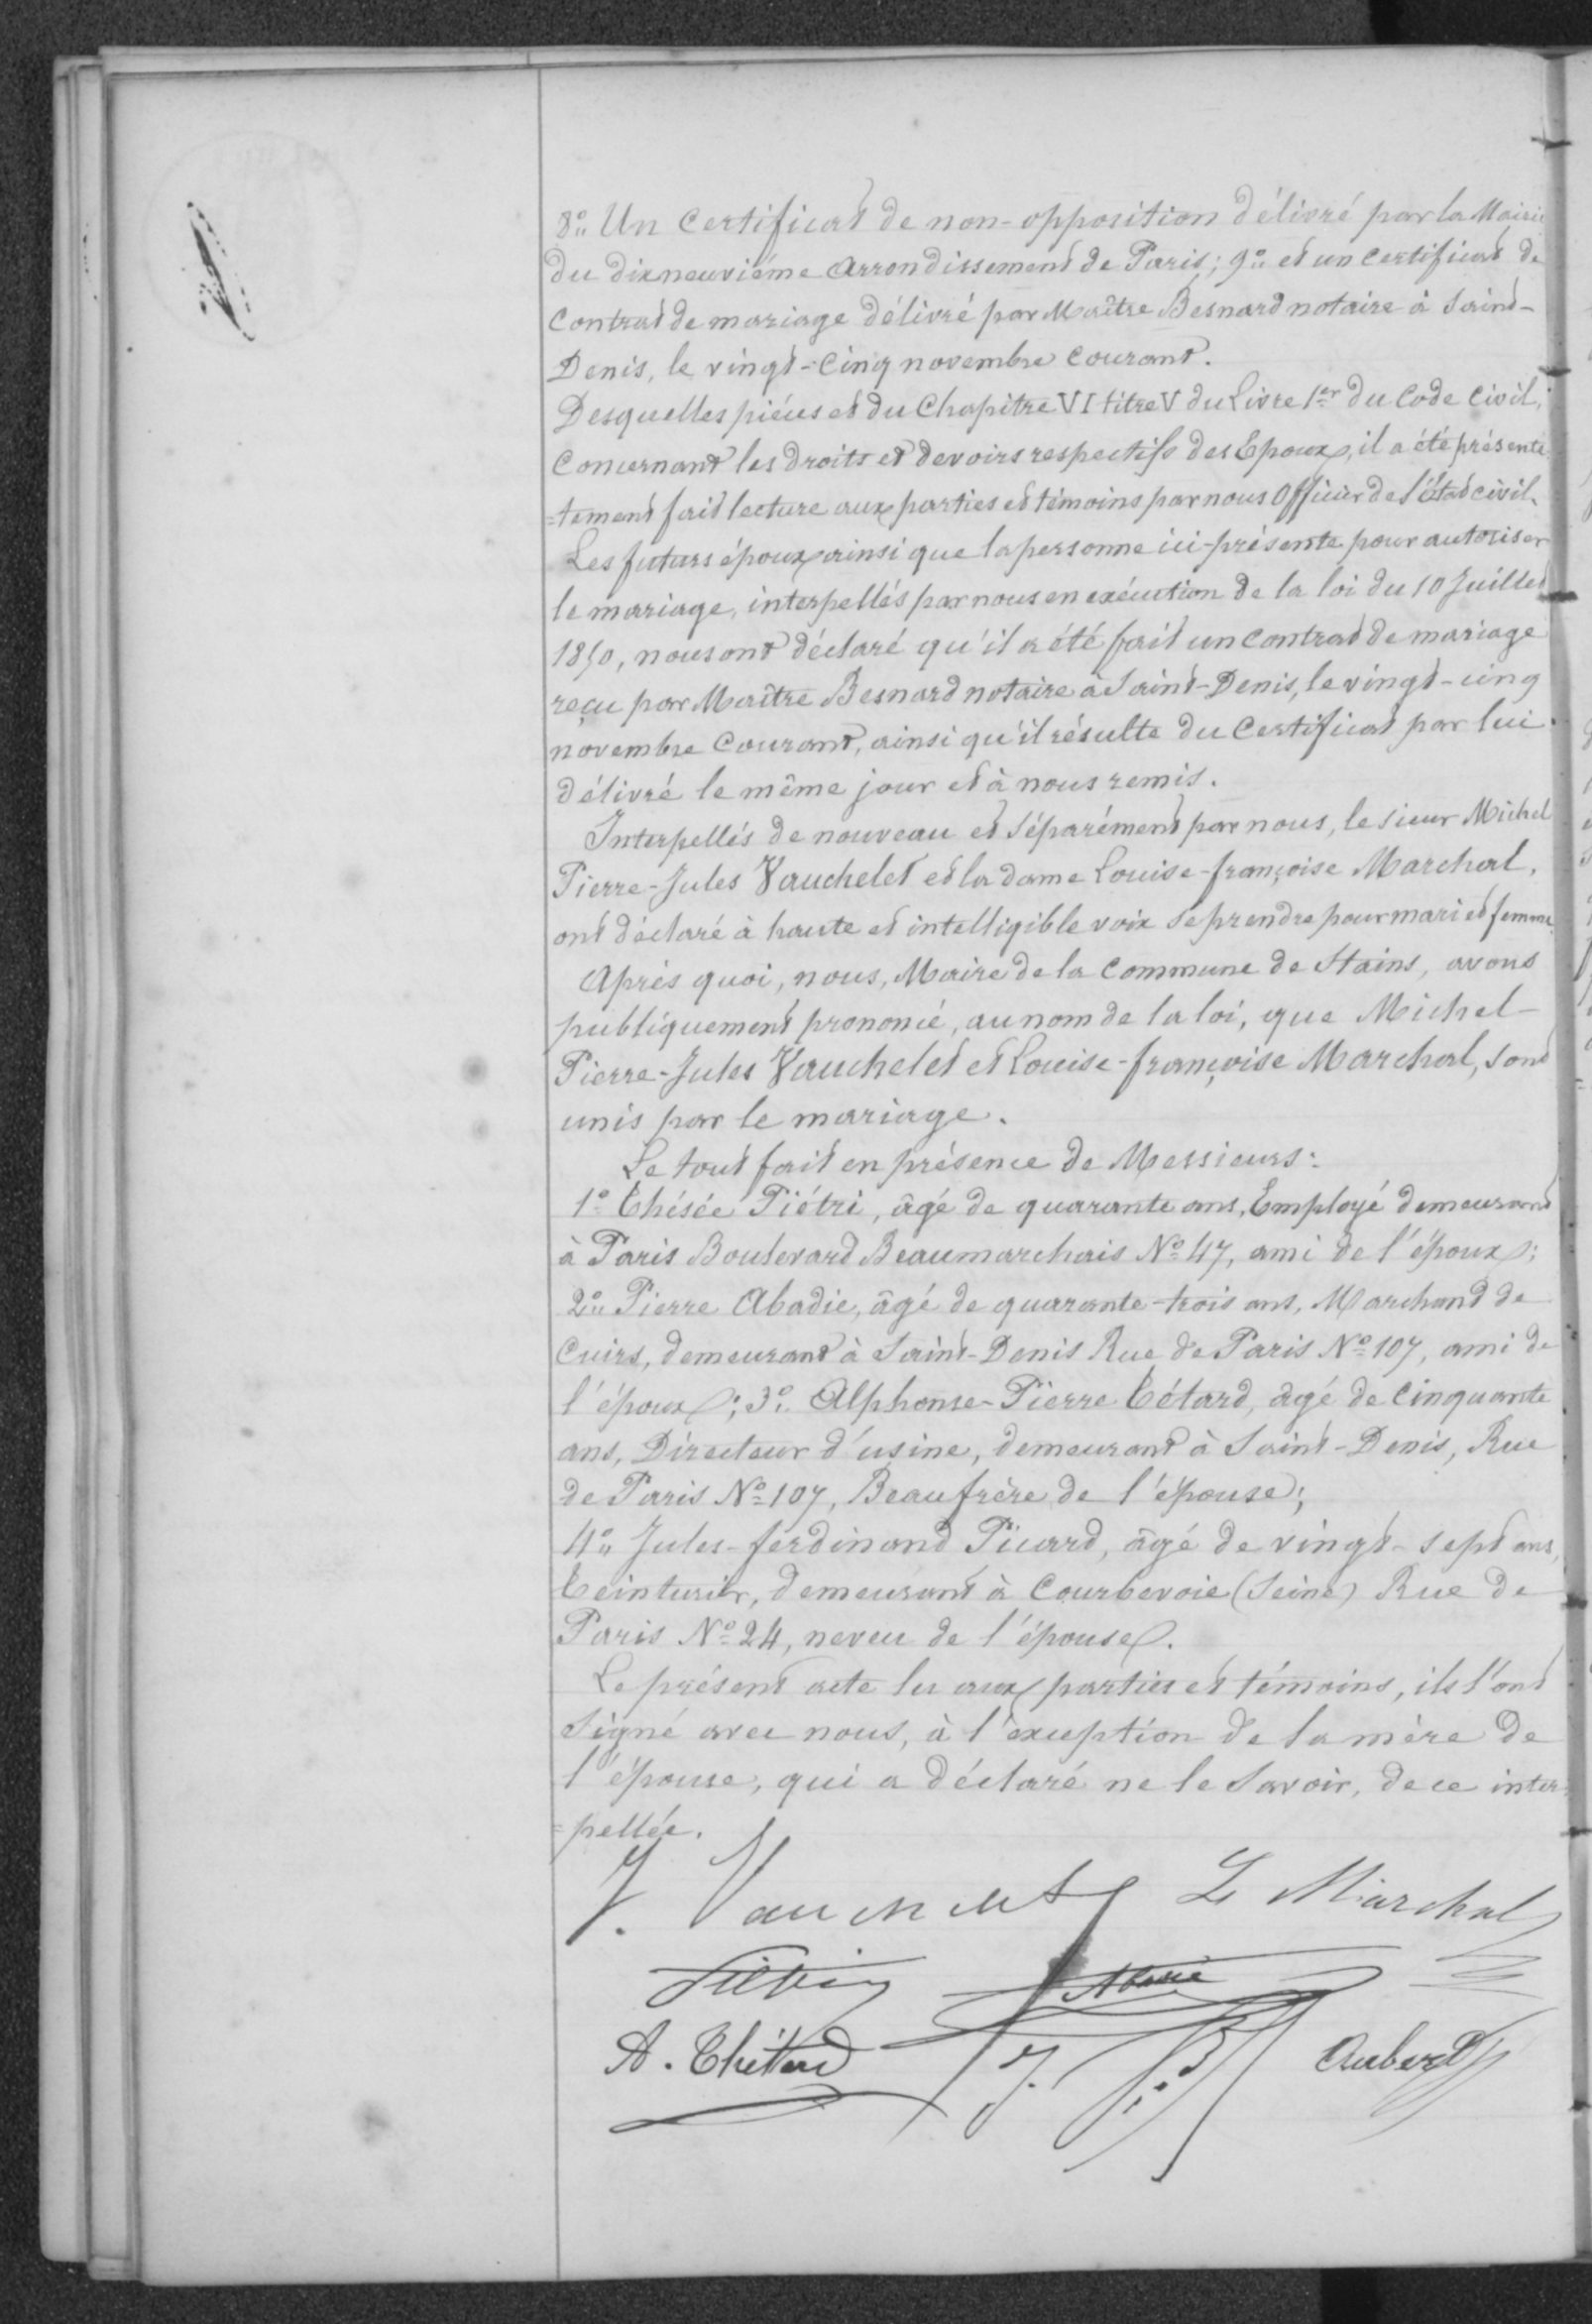8° Un certificat de non-opposition délivré par la Mairie
du dix-neuvième arrondissement de Paris; 9° et un certificat de
Contrat de mariage délivré par Maître Besnard notaire à Saint-
Denis, le vingt-cinq novembre courant.
Desquelles pièces et du Chapitre VI titre V du Livre 1er du Code Civil
Concernant les droits et devoirs respectifs des Epoux, il a été présente
-tement fait lecture aux parties et témoins par nous Officier de l'état-civil.
Les futurs époux ainsi que la personne ici-présente pour autoriser
le mariage interpellés par nous en exécution de la loi du 10 juillet
1850, nous ont déclaré qu'il a été fait un contrat de mariage
reçu par Maître Besnard notaire à Saint-Denis, le vingt-cinq
novembre Courant, ainsi qu'il résulte du Certificat par lui
délivré le même jour et à nous remis.
Interpellés de nouveau et séparément par nous, le sieur Michel
Pierre-Jules Vauchelet et la dame Louise-françoise Marchorl,
ont déclaré à haute et intelligible voix se prendre pour mari et femme.
Après quoi, nous, Maire de la Commune de Stains, avons
publiquement prononcé, au nom de la loi, que Michel
Pierre-Jules Vauchelet et Louise-françoise Marchorl, sont
unis par le mariage.
Le tout fait en présence de Messieurs:
1° Thésée Piétri, âgé de quarante ans, Employé demeurant
à Paris Boulevard Beaumarchais N°47, ami de l'époux;
2° Pierre Abadie, âgé de quarante trois ans, Marchand de
Cuirs, demeurant à Saint-Denis Rue de Paris N°107, ami de
l'époux; 3° Alphonse-Pierre tétard, âgé de cinquante
ans, Directeur d'usine, demeurant à Saint-Denis, Rue
de Paris N°107, Beaufrère de l'épouse;
4° Jules ferdinand Picard, âgé de vingt-sept ans
teinturier, demeurant à Courbevoie (Seine) Rue de
Paris N°24 neveu de l'épouse.
Le présent acte lu aux parties et témoins, ils ont
signé avec nous, à l'exception de la mère de
l'épouse, qui a déclaré ne le savoir, de ce inter-
-pellée.
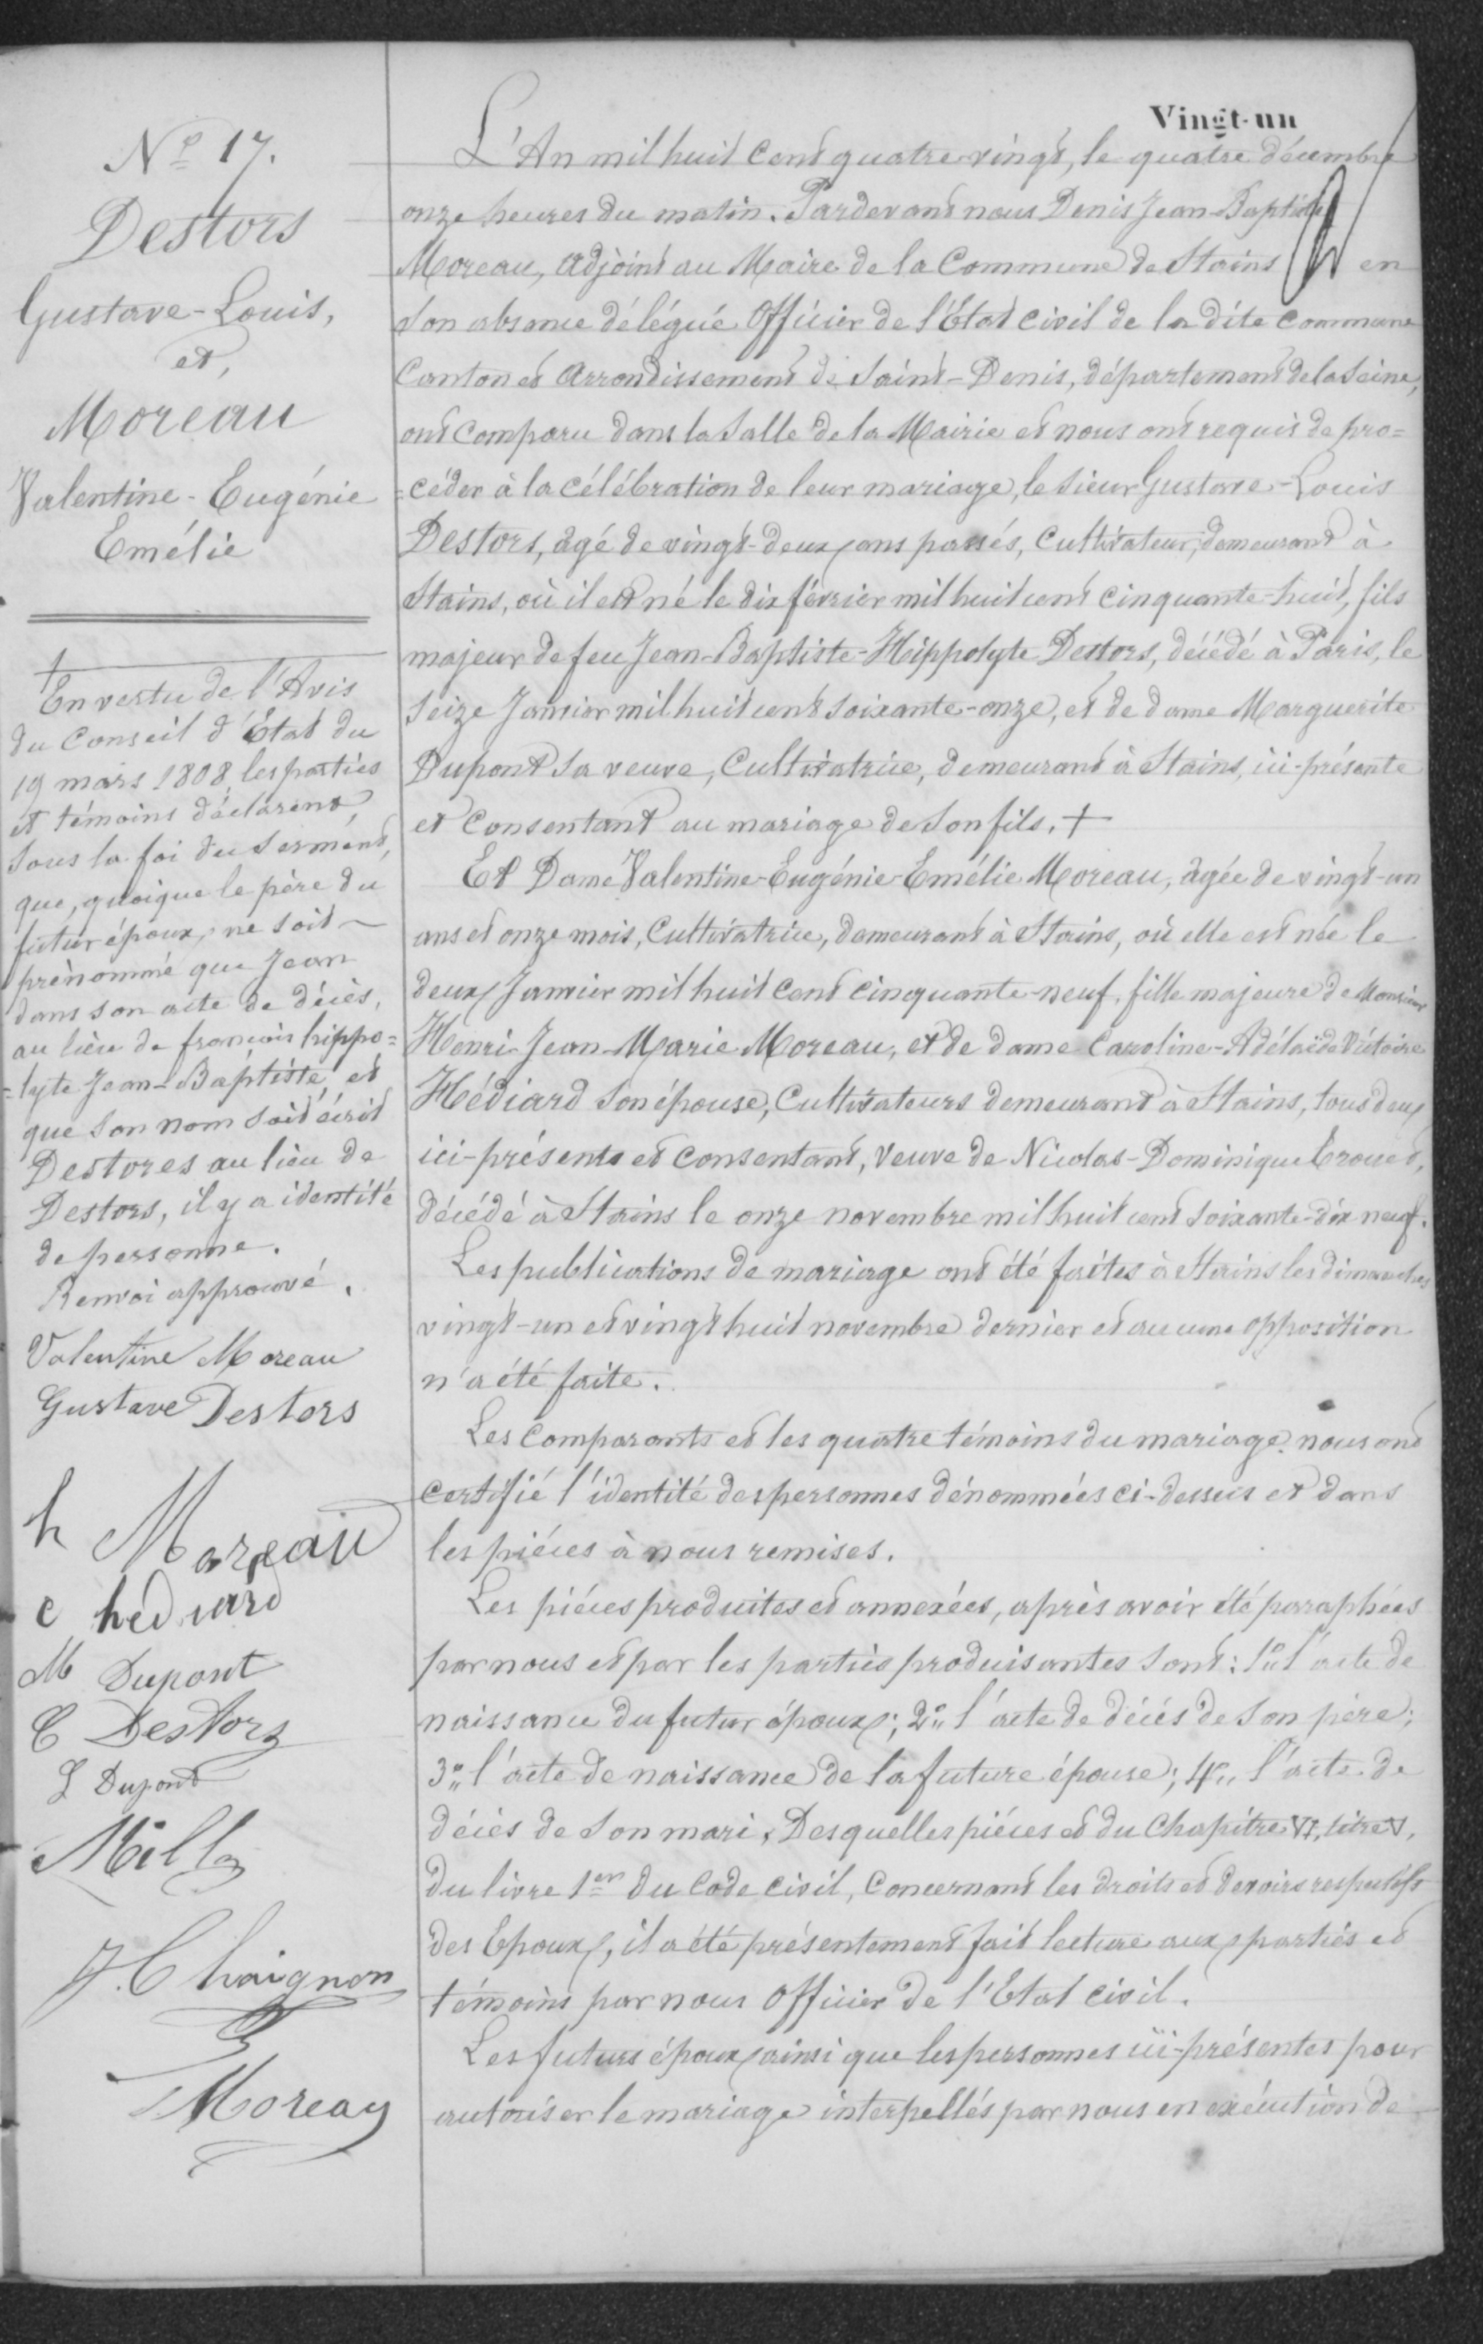N°17
Destors
Gustave-Louis
et
Moreau
Valentine-Eugénie
Emilie
L'An mil huit cent quatre vingt, le quatre décembre
onze heures du matin. Par devant nous Denis Jean-Baptiste
Moreau, Adjoint au Maire de la Commune de Stains en
son absence délégué Officier de l'Etat Civil de la dite Commune
Canton et arrondissement de Saint-Denis, département de la Seine,
ont comparu dans la Salle de la Mairie et nous ont requis de pro-
céder à la célébration de leur mariage, le sieur Gustave-Louis
Destors, agé de vingt-deux ans passés, cultivateur, demeurant à
Stains, où il est né le dix février mil huit cent cinquante-huit, fils
majeur de feu Jean-Baptiste-Hippolyte Destors, décédé à Paris, le
seize Janvier mil huit cent soixante-onze, et de dame Marguerite
Dupont, sa veuve, Cultivatrice, demeurant à Stains, ici-présente
et consentant au mariage de son fils.
Et Dame Valentine-Eugénie-Emélie Moreau, âgée de vingt-un
ans et onze mois, Cultivatrice, demeurant à Stains, où elle est née le
deux Janvier mil huit cent cinquante-neuf, fille majeure de Monsieur
Henri Jean-Marie Moreau, et de dame Caroline Adélaïde Victoire
Hédiard son épouse, Cultivateurs demeurant à Stains, tous deux
ici-présents et consentant, Veuve de Nicolas-Dominique troués
décédé à Stains le onze novembre mil huit cent soixante-dix neuf.
Les publications de mariage ont été faites à Stains les dimanches
vingt-un et vingt-huit novembre dernier et aucune opposition
n'a été faite.
Les comparants et les quatre témoins du mariage nous ont
certifié l'identité des personnes dénommées ci-dessus et dans
les piéces à nous remises.
Les piéces produites et annexées, après avoir été paraphées
par nous et par les parites produisantes sont: 1° l'acte de
naissance du futur époux; 2° l'acte de décès de son père;
3° l'acte de naissance de la future épouse; 4° l'acte de
décès de son mari, Desquelles piéces et du Chapitre VI livre V
du livre 1er du Code Civil, concernant les droits et le devoirs respectifs
des Epoux, il a été présentement fait lecture aux parties et
témoins par nous Officier de l'Etat civil.
Les futurs époux ainsi que les personnes ici-présentes pour
autoriser le mariage interpellés par nous en exécution de